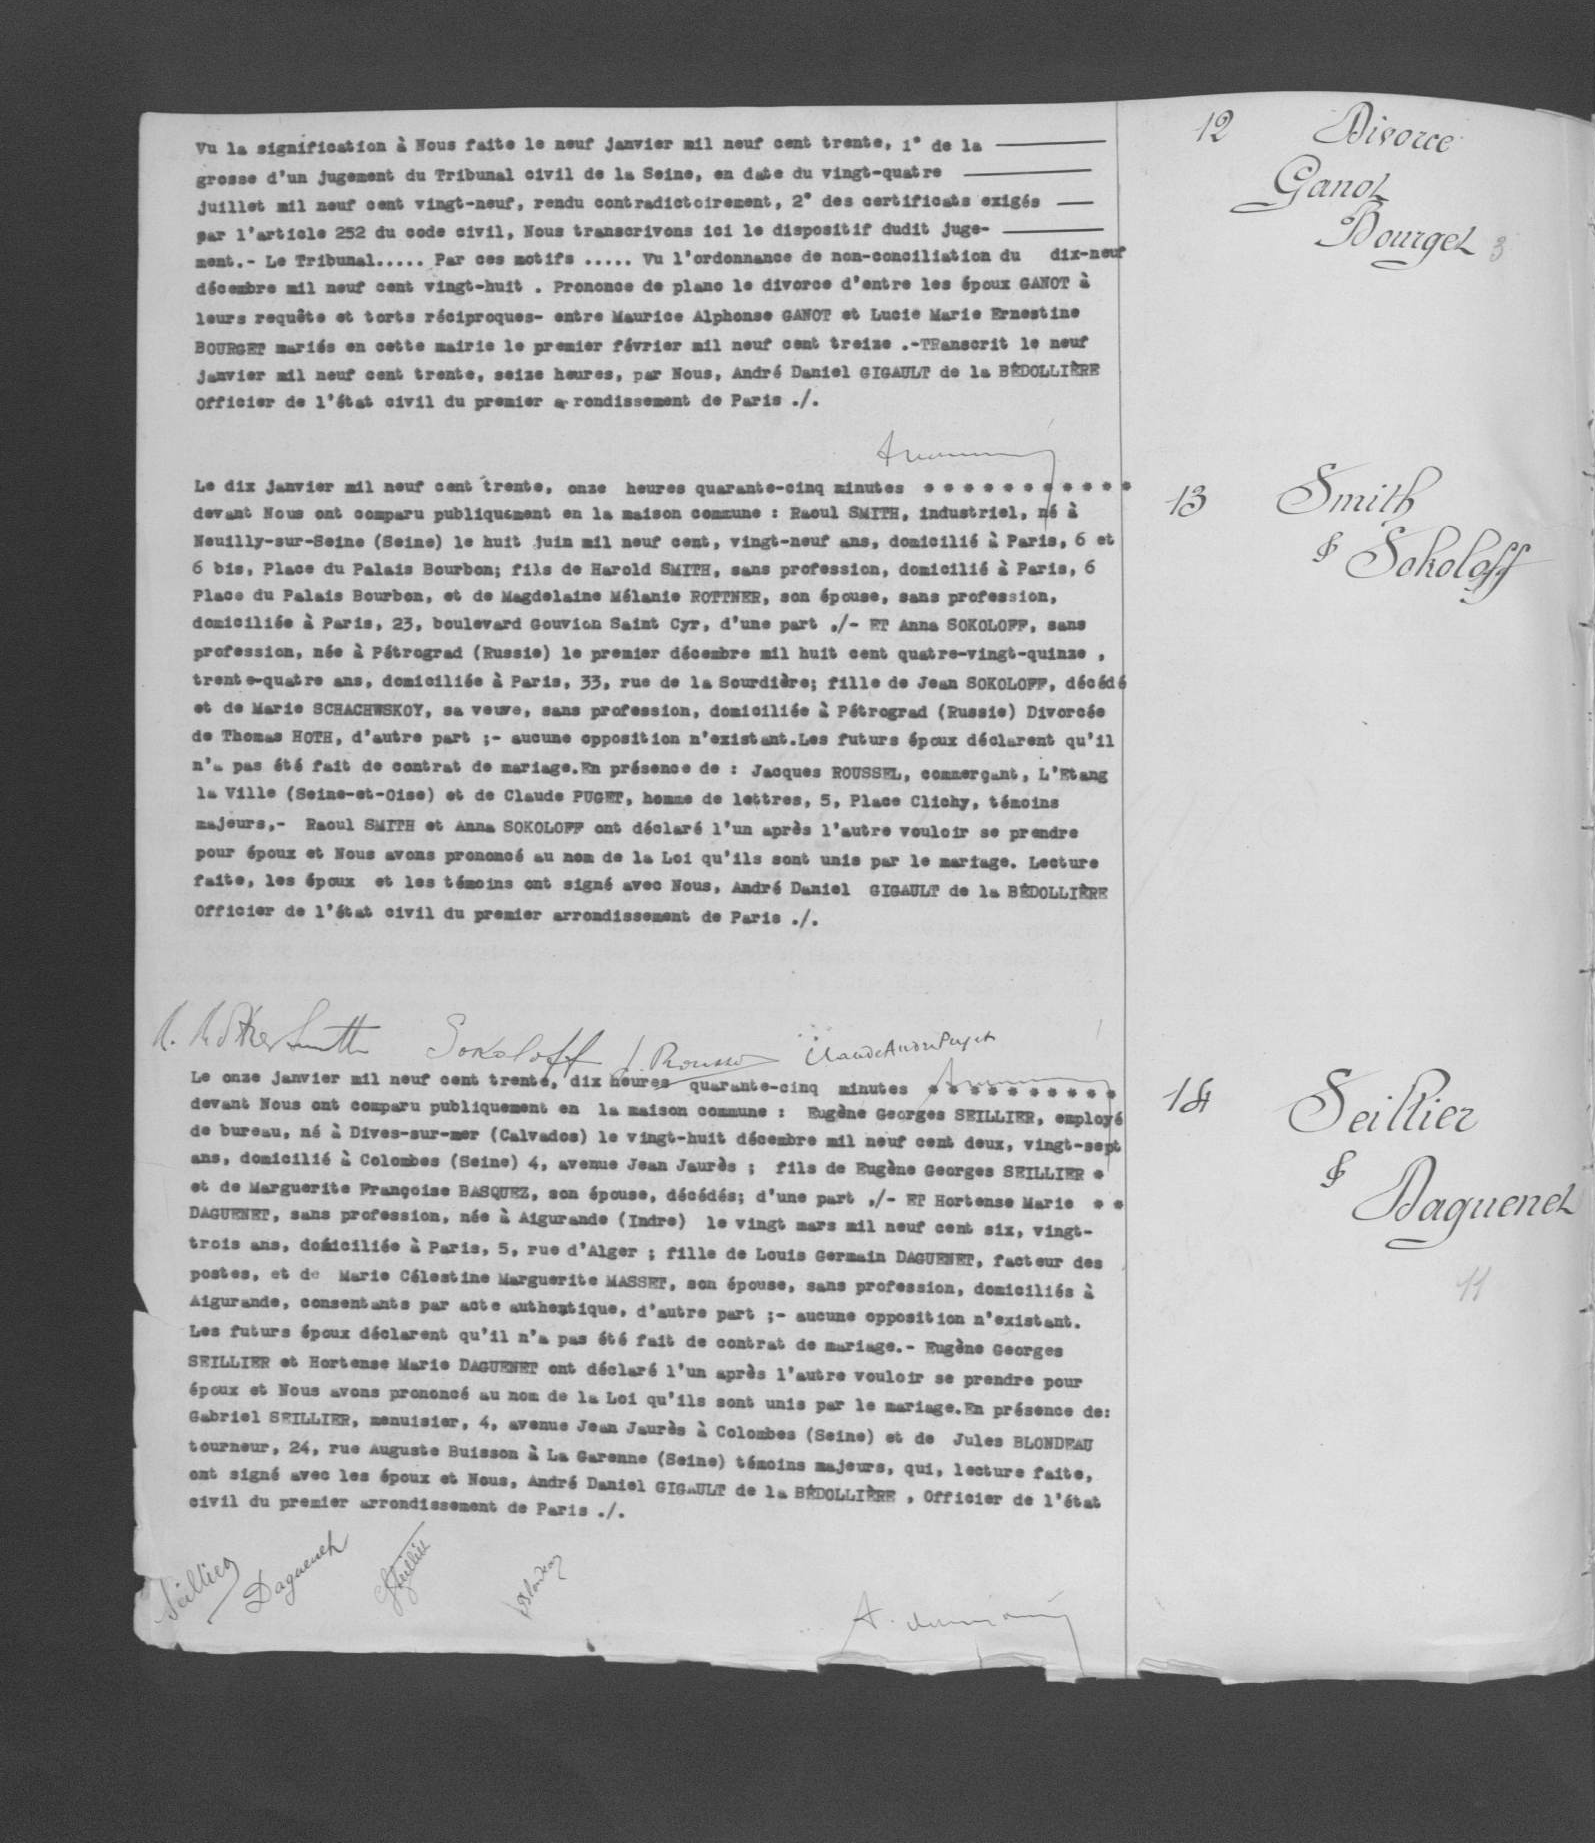Vu la signification à Nous faite le neuf janvier mil neuf cent trente, 1° de la -
grosse d'un jugement du Tribunal civil de la Seine, en date du vingt-quatre -
juillet mil neuf cent vingt-neuf, renu contradictoirement, 2° des certificats exigés -
par l'article 252 du code civil, Nous transcrivons ici le dispositif dudit juge-
ment .-Le Tribunal ..... Par ces motifs ..... Vu l'ordonnance de non-conciliation du dix-neuf
décembre mil neuf cent vingt-huit. Prononce de plano le divorce d'entre les époux GANOT à
leurs requête et torts réciproques-entre Maurice Alphonse GANOT et Lucie Marie Ernestine
BOURGET mariés en cette mairie le premier février mil neuf cent treize .-Transcrit le neuf
janvier mil neuf cent trente, seize heures, par Nous, André Daniel GIGAULT de la BEDOLLIERE
Officier de l'état civil du premier arrondissement de Paris ./.
Le dix janvier mil neuf cent trente onze heures quarante-cinq minutes ****
devant Nous ont comparu publiquement en la maison commune: Raoul SMITH, industriel, né à
Neuilly-sur-Seine (Seine) le huit juin mil neuf cent, vingt-neuf ans, domicilié à Paris, 6 et
6 bis, Place du Palais Bourbon; fils de Harold SITH, sans profession, domicilié à Paris, 6
Place du Palais Bourbon, et de Magdelaine Mélanie ROTTNER, son épouse, sans profession,
domiciliée à Paris, 23, boulevard Gouvion Saint-Cyr, d'une part ./. ET Anna SOKOLOFF, sans
profession, née à Pétrograd (Russie) le premier décembre mil huit cent quatre-vingt-quinze,
trente-quatre ans, domiciliée à Paris, 33, rue de la Sourdière; fille de Jean SOKOLOFF, décédé
et de Marie SCHACHWSKOY, sa veuve, sans profession, domiciliée à Pétrograd (Russie) Divorcée
de Thomas HOYH, d'autre part; aucune opposition n'existant. Les futurs époux déclarent qu'il
n'a pas été fait de contrat de mariage. En présence de: Jacques ROUSSEL, commerçant, l'Etang
la Ville (Seine-et-Oise) et de Claude PUGET, homme de lettres, 5, Place Clichy, témoins
majeurs .-Raoul SMITH et Anna SOKOLOFF ont déclaré l'un après l'autre vouloir se prendre
pour époux et Nous avons prononcé au nom de la Loi qu'ils sont unis par le mariage. Lecture
faite, les époux et les témoins ont signé avec Nous, André Daniel GIGAULT de la BEDOLLIERE
Officier de l'état civil du premier arrondissement de Paris ./.
Le onze janvier mil neuf cent trente, dix heures quarante-cinq minutes ****
devant Nous ont comparu publiquement en la maison commune: Eugène Georges SEILLIER, employé
de bureau, né à Dives-sur-mer (Calvados) le vingt-huit décembre mil neuf cent deux, vingt-sept
ans, domicilié à Colombes (Seine) 4, avenue Jean Jaurès; fils de Eugène George SEILLIER *
et de Marguerite Françoise BASQUEZ, son épouse, décédés; d'une part ./-ET Hortense Marie * *
DAGUENET, sans profession, née à Aiguerande (Indre) le vingt mars mil neuf cent six, vingt-
trois ans, domiciliée à Paris, 5, rue d'Alger; fille de Louis Germain DAGUENET, facteur des
postes, et de Marie Célestine Marguerite MASSET, son épouse, sans profession, domiciliés à
Aigurande, consentants par cette authentique, d'autre part ;-aucune opposition n'existant.
Les futurs époux déclarent qu'il n'a pas été fait de contrat de mariage .-Eugène Georges
SEILLIER et Hortense Marie DAGUENET ont déclaré l'un après l'autre vouloir se prendre pour
époux et Nous avons prononcé au nom de la Loi qu'ils sont unis par le mariage. En présence de:
Gabriel SEILLIER, menuisier, 4, avenue Jean Jaurès à Colombes (Seine) et de Jules BLONDEAU
tourneur, 24, rue Auguste Buisson à la Garenne (Seine) témoins majeurs, qui, lecture faite,
ont signé avec les époux et Nous, André Daniel GIGAULT de la BEDOLLIERE, Officier de l'état
civil du premier arrondissement de Paris ./.
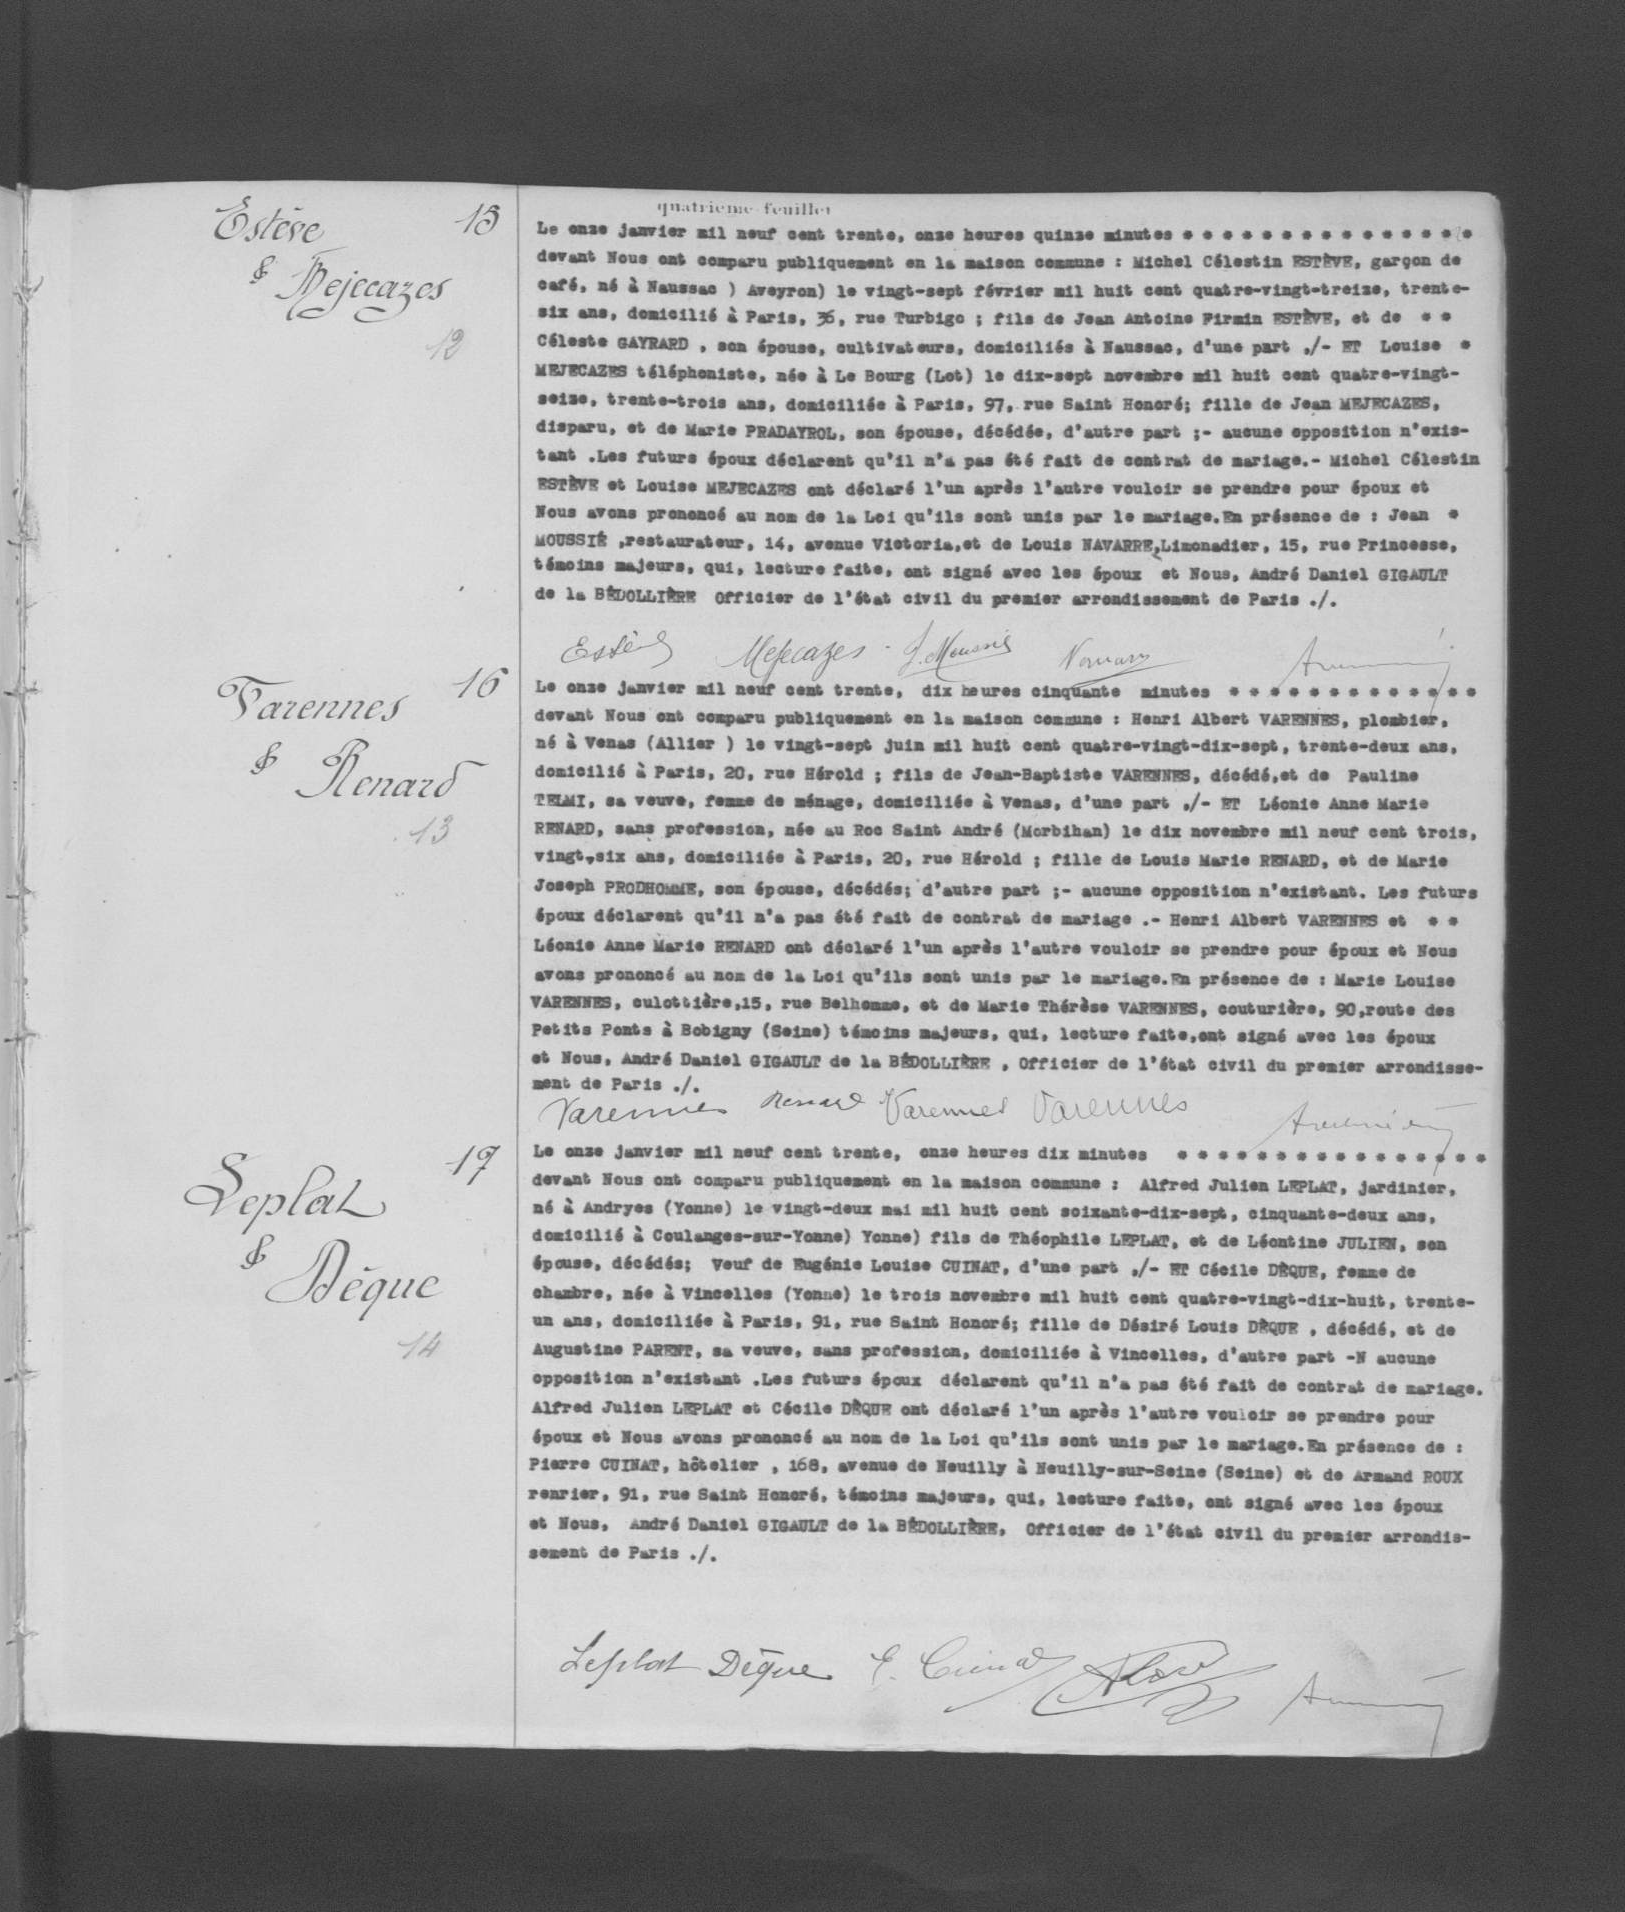Le onze janvier mil neuf cent trente, onze heures quinze minutes ****
devant Nous ont comparu publiquement en la maison commune: Michel Célestin ESTEVE, garçon de
café, né à Naussac) Aveyron) le vingt-sept février mil huit cent quatre-vingt-treize, trente-
six ans, domicilié à Paris, 36, rue Turbigo; fils de Jean Antoine Firmin ESTEVE, et de * *
Céleste GAYRARD, son épouse, cultivateurs, domiciliés à Naussac, d'une part ./-ET Louise *
MEJECAZES téléphoniste, née à Le Bourg (Lot) le dix-sept novembre mil huit cent quatre-vingt-
seize, trente-trois ans, domiciliée à Paris, 97, rue Saint Honoré; fille de Jean MEJECAZES,
disparu, et de Marie PRADAYROL, son épouse décédée, d'autre part ;-aucune opposition n'exis-
tant. Les futurs époux déclarent qu'il n'a pas été fait de contrat de mariage .-Michel Célestin
ESTEVE et Louise MEJECAZES ont déclaré l'un après l'autre vouloir se prendre pour époux et
Nous avons prononcé au nom de la Loi qu'ils sont unis par le mariage. En présence de: Jean *
MOUISSIE, restaurateur, 14, avenue Victoria, et de Louis NAVARRE, Limonadier, 15, rue Princesse,
témoins majeurs, qui lecture faite, ont signé avec les époux et Nous, André Daniel Gigault
de la BEDOLLIERE Officier de l'état civil du premier arrondissement de Paris ./.
Le onze janvier mil neuf cent trente, dix heures cinquante minutes ****
devant Nous ont comparu publiquement en la maison commune: Henri Albert VARENNES, plombier,
né à Venas (Allier) le vingt-sept juin mil huit cent quatre-vingt-dix-sept, trente-deux ans,
domicilié à Paris, 20, rue Hérold; fils de Jean-Baptiste VARENNES, décédé, et de Pauline
TELMI, sa veuve, femme de ménage, domiciliée à Venas, d'une part ./-ET Léonie Anne Marie
RENARD, sans profession, née au Roc Saint André (Morbihan) le dix novembre mil neuf cent trois,
vingt-six ans, domiciliée à Paris, 20, rue Hérold; fille de Louis Marie RENARD, et de Marie
Joseph PROGHOMME, son épouse, décédés; d'autre part; aucune opposition n'existant. Les futurs
époux déclarent qu'il n'a pas été fait de contrat de mariage .-Henri Albert VARENNES et * *
Léonie Anne Marie RENARD ont déclaré l'un après l'autre vouloir se prendre pour époux et Nous
avons prononcé au nom de la Loi qu'ils sont unis par le mariage. En présence de: Marie Louise
VARENNES, culottière, 15, rue Belhomme, et de Marie Thérèse VARENNES, couturière, 90, route des
Petits Ponts à Bobigy (Seine) témoins majeurs, qui, lecture faite, ont signé avec les époux
et Nous, André Daniel GIGAULT de la BEDOLLIERE, Officier de l'état civil du premier arrondisse-
ment de Paris ./.
Le onze janvier mil neuf cent trente, onze heures dix minutes ****
devant Nous ont comparu publiquement en la maison commune: Alfred Julien LEPLAT, jardinier,
né à Andryes (Yonne) le vingt-deux mai mil huit cent soixante-dix-sept, cinquante-deux ans,
domicilié à Coulanges-sur-Yonne) Yonne) fils de Théophile LEPLAT, et de Léontine JULIEN, son
épouse, décédés; Veuf de Eugénie Louise CUINAT, d'une part ./-ET Cécile DEQUE, femme de
chambre, née à Vincelles (Yonne) le trois novembre mil huit cent quatre-vingt-dix-huit, trente-
un ans, domiciliée à Paris, 91, rue Saint Honoré; fille de Désiré Louis DEQUE, décédé, et de
Augustine PARENT, sa veuve, sans profession, domiciliée à Vincelles, d'autre part. N aucune
opposition n'existant. Les futurs époux déclarent qu'il n'a pas été fait de contrat de mariage.
Alfred Julien LEPLAT et Cécile DEQUE ont déclaré l'un après l'autre vouloir se prendre pour
époux et Nous avons prononcé au nom de la Loi qu'ils sont unis par le mariage. En présence de:
Pierre CUINAT, hôtelier, 168, avenue de Neuilly à Neuilly-sur-Seine (Seine) et de Armand ROUX
renrier, 91, rue Saint Honoré, témoins majeurs, qui, lecture faite, ont signé avec les époux
et Nous, André Daniel GIGAULT de la BEDOLLIERE, Officier de l'état civil du premier arrondis-
sement de Paris ./.